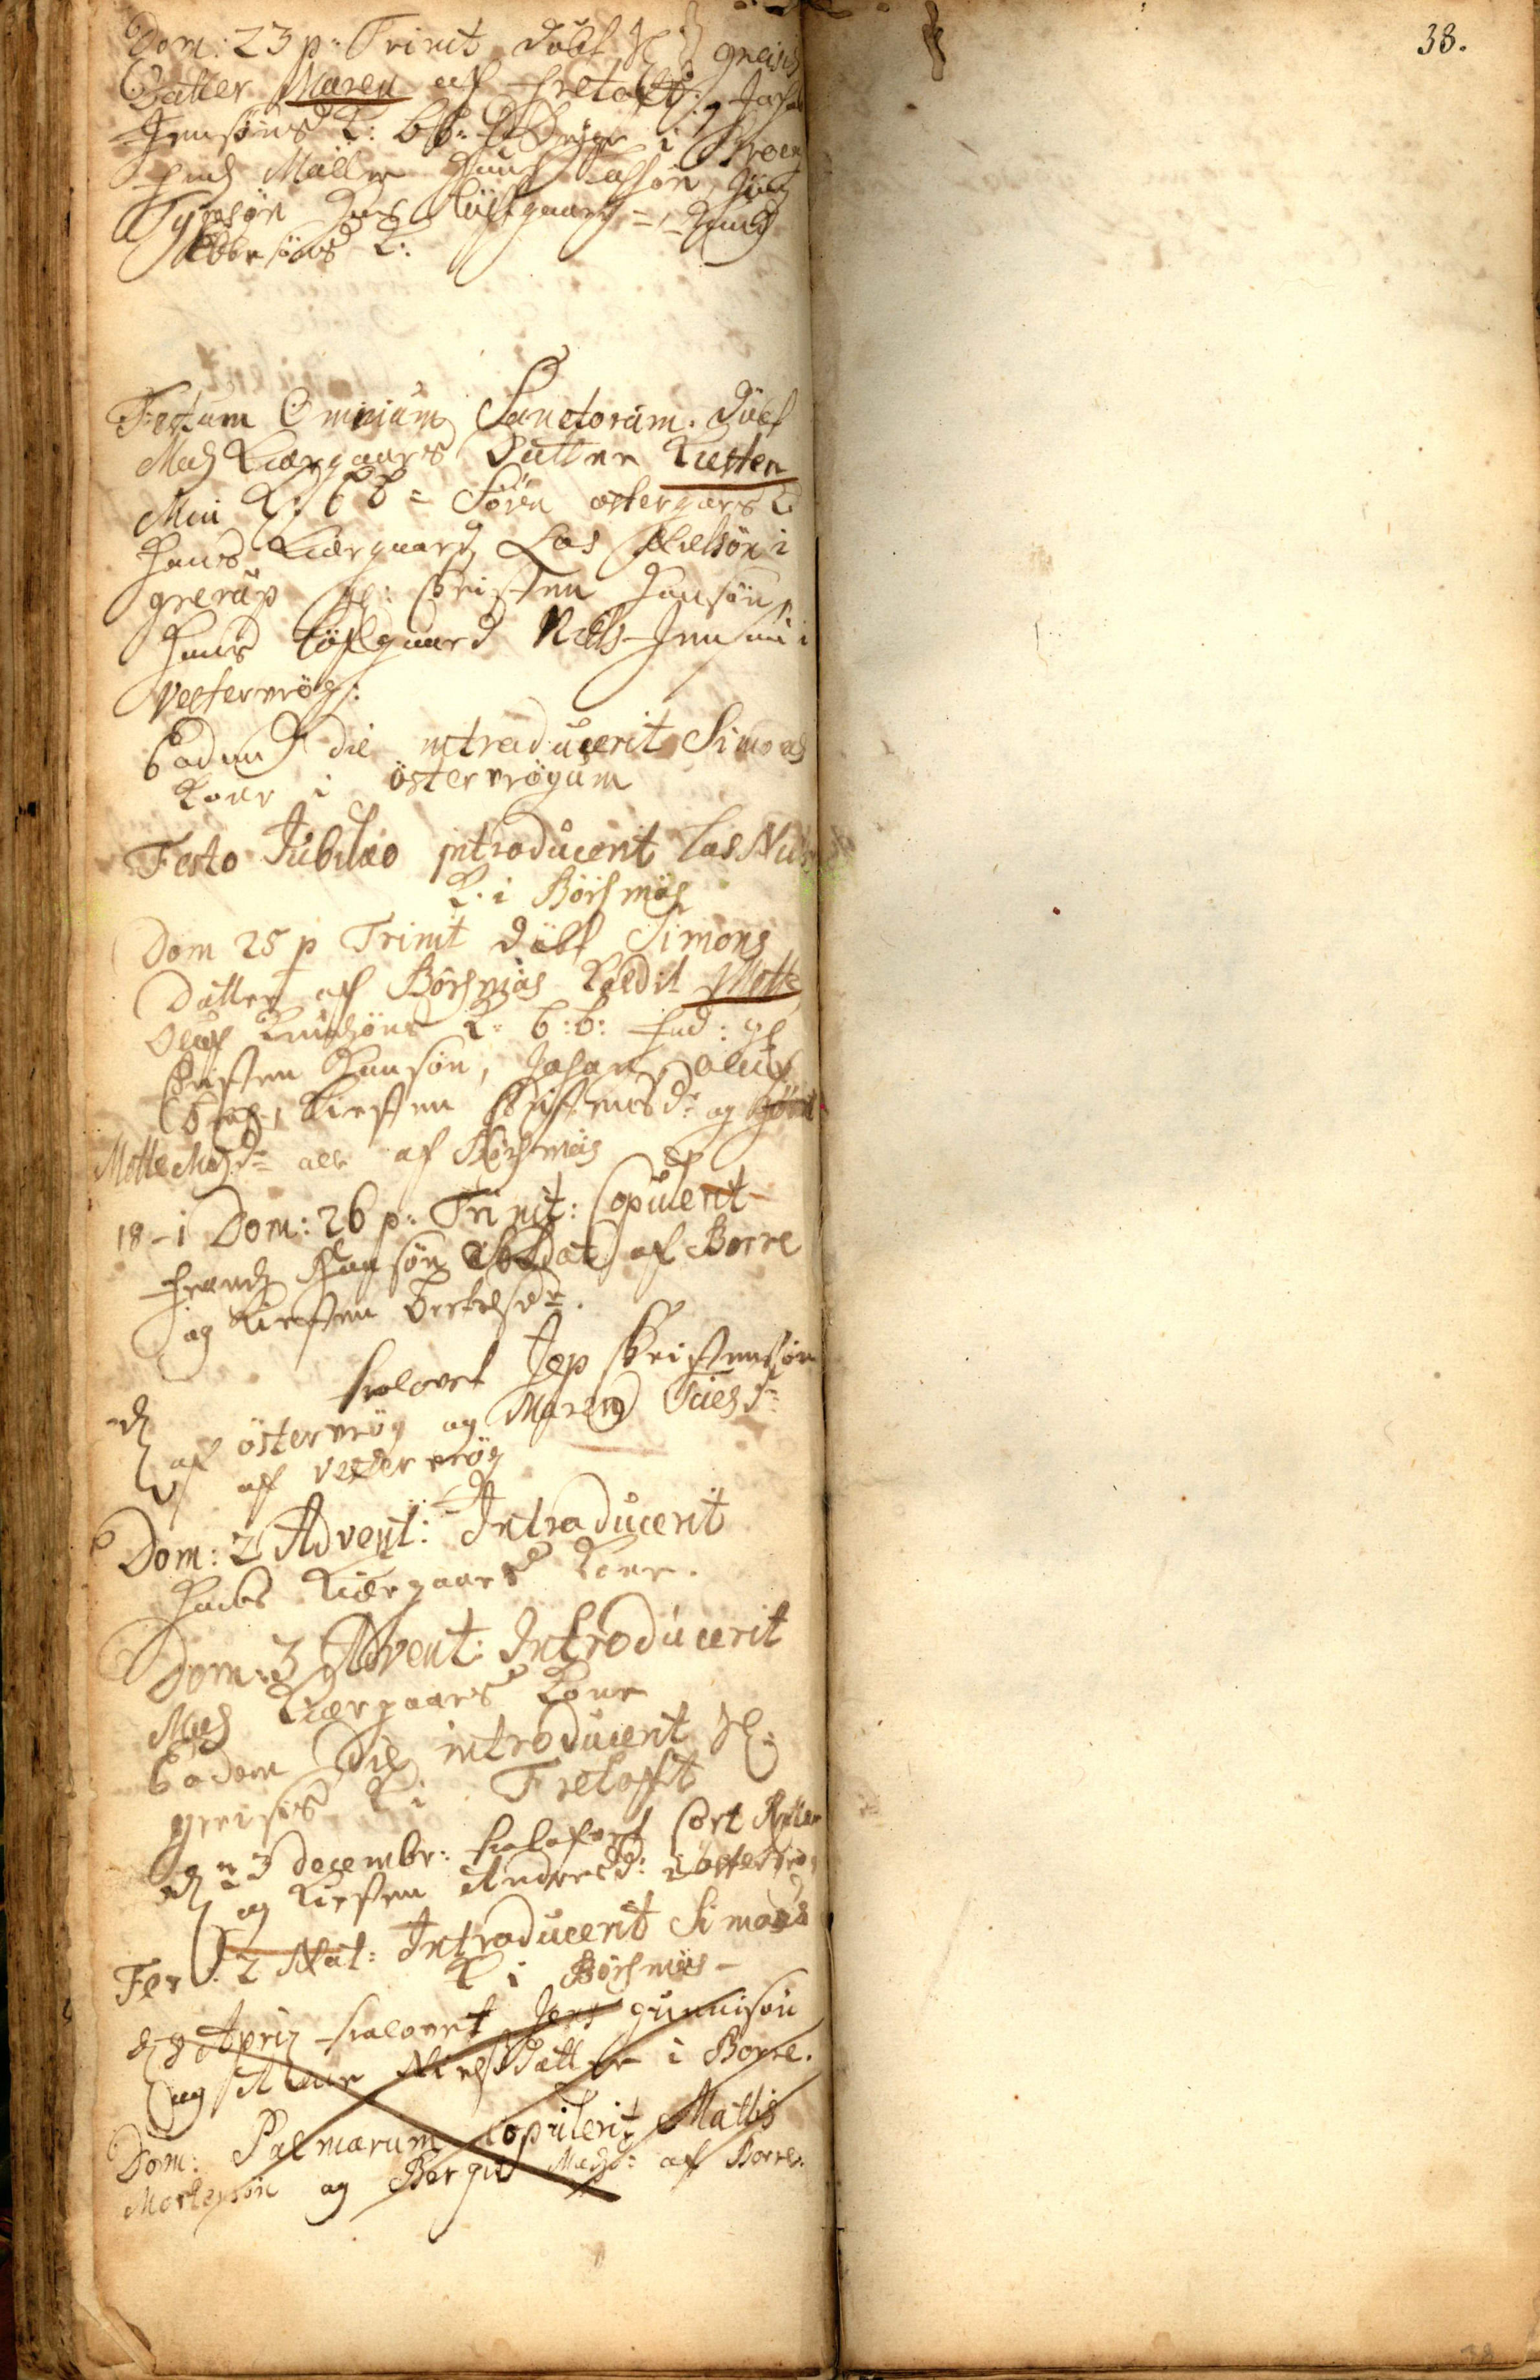Dom: 23 p: Trinit døbt Sl ## Greisis
Datter Maren af Fretoft: Jachob
Jensøns K: bb: Byrge i Broeng
Frandz Møller Hans Lassøn Jørg
Tygesøn Hans Løftgaard Hans
Ebbesøns K:
Festum Omnium Sanctorum Døbt
Madz Kiærgaars Datter Kiesten
Min K: bb= Søren Østergaars K
Hans Kiærgaard, Las Nielsøn i
Grerup gl. Christen Hansøn
Hans Løftgaard, Niels Jensøn i
Vestervrøg:
Eodem Die introducerit Simons
Kone i Østervrøgum
Festo Jubilæo introducerit Las Nielsens
K. i Børsmøs
Dom 25 p Trinit døbt Simons
Datter af Børsmos kaldit Mette
Oluf Knudzøns K: b:b: Fad: gl
Christen Hansøn Johan, Oluf
Brich, Kirsten Christensdr og ##
Mette Madzdr alle af Børsmøs
18-i Dom: 26 p: Trinit: Copulerit
Frandz Hansøn Soldat af Borre
og Kirsten Bertelsdr.
D
trolovet Jep Christensøn
af Østervrøg og Maren Tuesdr
af Vestervrøg
Dom: 2 Advent: Introducerit
Hans Kiærgaars Kone.
Dom: 3 Advent: Introducerit
Madz Kiærgaars Kone
Eodem Die introducerit Sl:
Greisis K i Fretoft
D 23 Decembr: trolofvet Cort Rytter
og Kiesten Andersdr af Østervrøg
Fer: 2 Nat: Introducerit Simons
K i Børsmøs-
D 8 April trolovet Jens Gunnisøn
og Anne## Nielsdatter i Borre.
Dom: Palmarum copulerit Mattis
Mortensøn og Bergit Madzdr af Borre.
38.
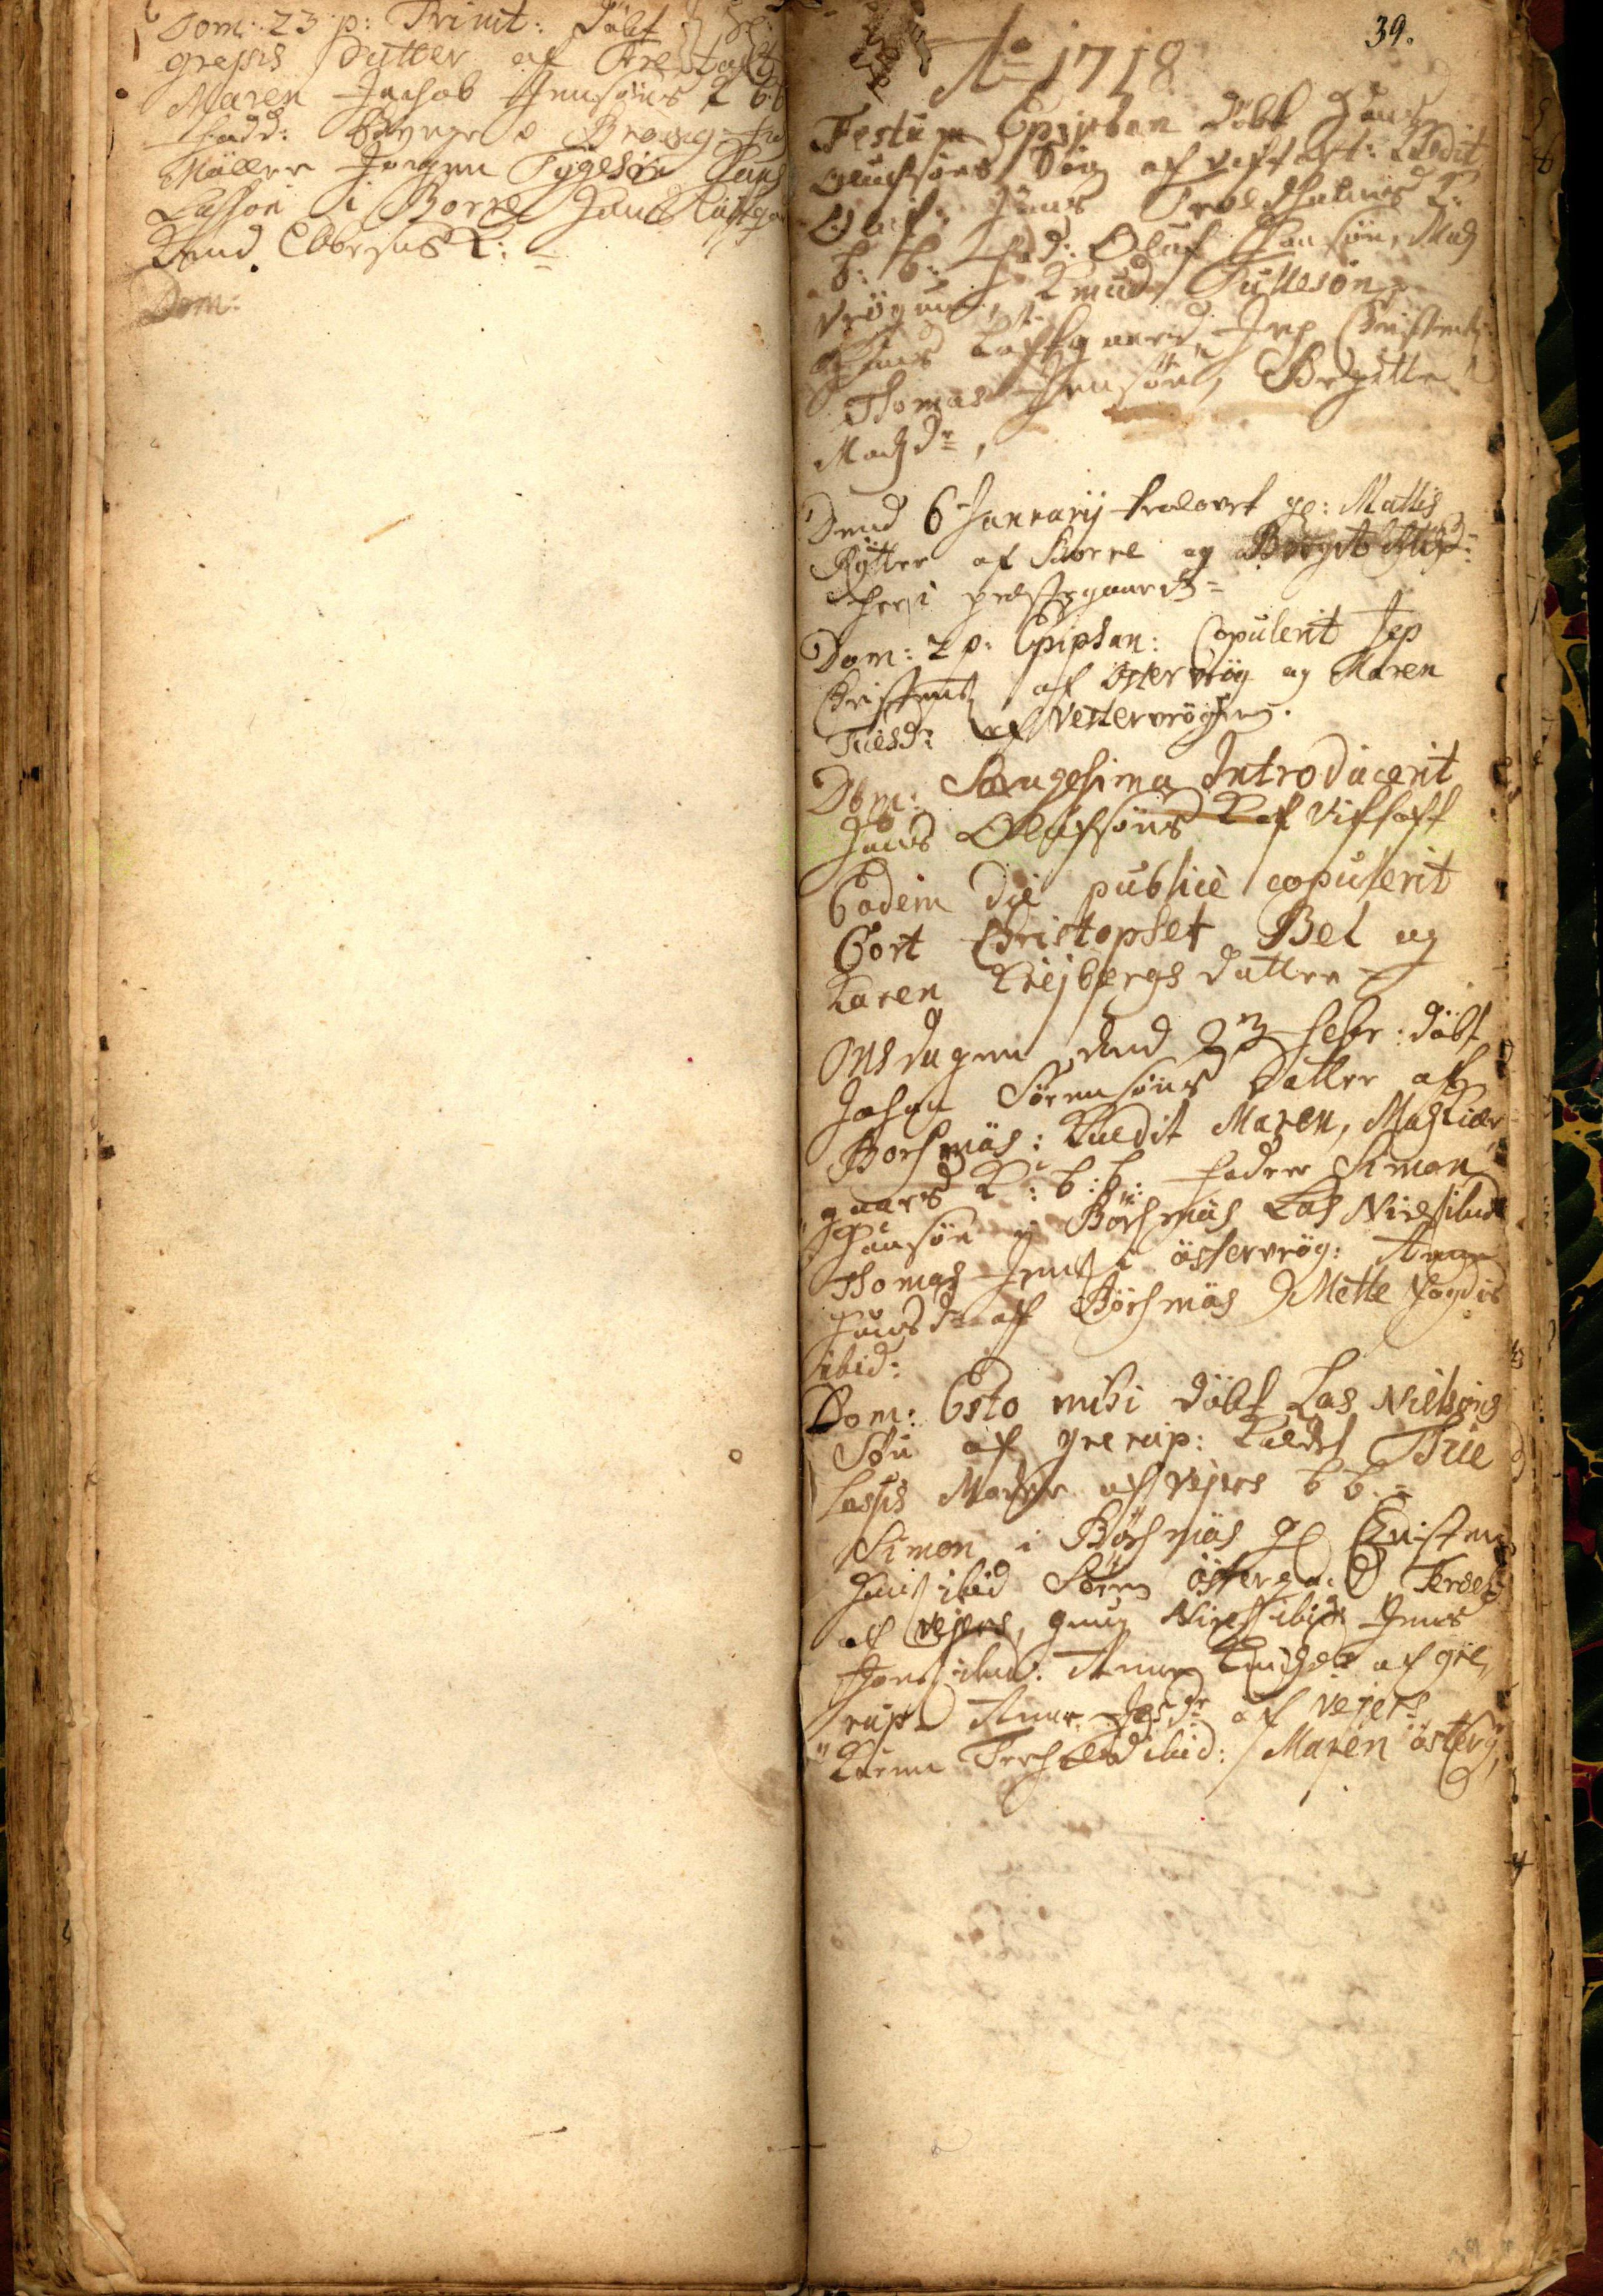Dom: 23 p: døbt ## Sl
Grejsis Datter af Fretoft
Maren- Jachob Jensøns K b.b
Fadd: Byrge i Broeng Fr##
Møller Jorgen Tygesøn Hans
Lassøn i Borre Hans ##
Knud Ebbesens:=
Dom:
39
39
Ao 1718
Festum Epiphan døbt Hans
Olufsøns Søn af Viftoft: kaldit
Oluf: Hans Troldholms K:
b: b: Fad: Oluf Hansøn, Madz
Vrøgum, Knud Tullesøn,
Hans Løftgaard, Jep Christens:
Thomas Jensøn, Bergitte
Madzdr.
Dend 6 Januarii trolovet gl. Mattis
Rytter af Borre og Bergit Madzd
her i Præstegaard=
Dom: 2 p: Epiphan: Copulerit Jep
Christens af Østervrøg og Karen
Tuesdr af Vestervrøgum.
Dom. Sexagesima Introducerit
Hans Olufsøns K af Viftoft
Eoedem Die publice copulerit
Cort Christopher Bel og
Karen Krejbergs Datter=
Onsdagen dend 23 Febr: døbt
Johan Sørensøns Datter af
Borsmøs: kaldit Maren, Madz Kiær¬
gaards K: b:b: Fadere Simon
Hansøn i Børsmøs, Las Niels ibid
Thomas Jens i Østervrøg: Anne
Hansdr af Børsmøs Mette Fogdis
ibid:
Dom: Esto Mihi døbt Las Nielsøns
Søn af Grerup: kaldet Thue
Lassis Moder af Vejers bb.=
Simon i Børsmos gl Christen
Hans ibid Søren Østergaard Terchel
af Vejers, Gunj Niels ibid, Jens
Jons ibid: Anne Knudzdr af Gre¬
rup, Anne Jesdr af Vejers,
Karen Terchelsd ibid Maren Østerg,